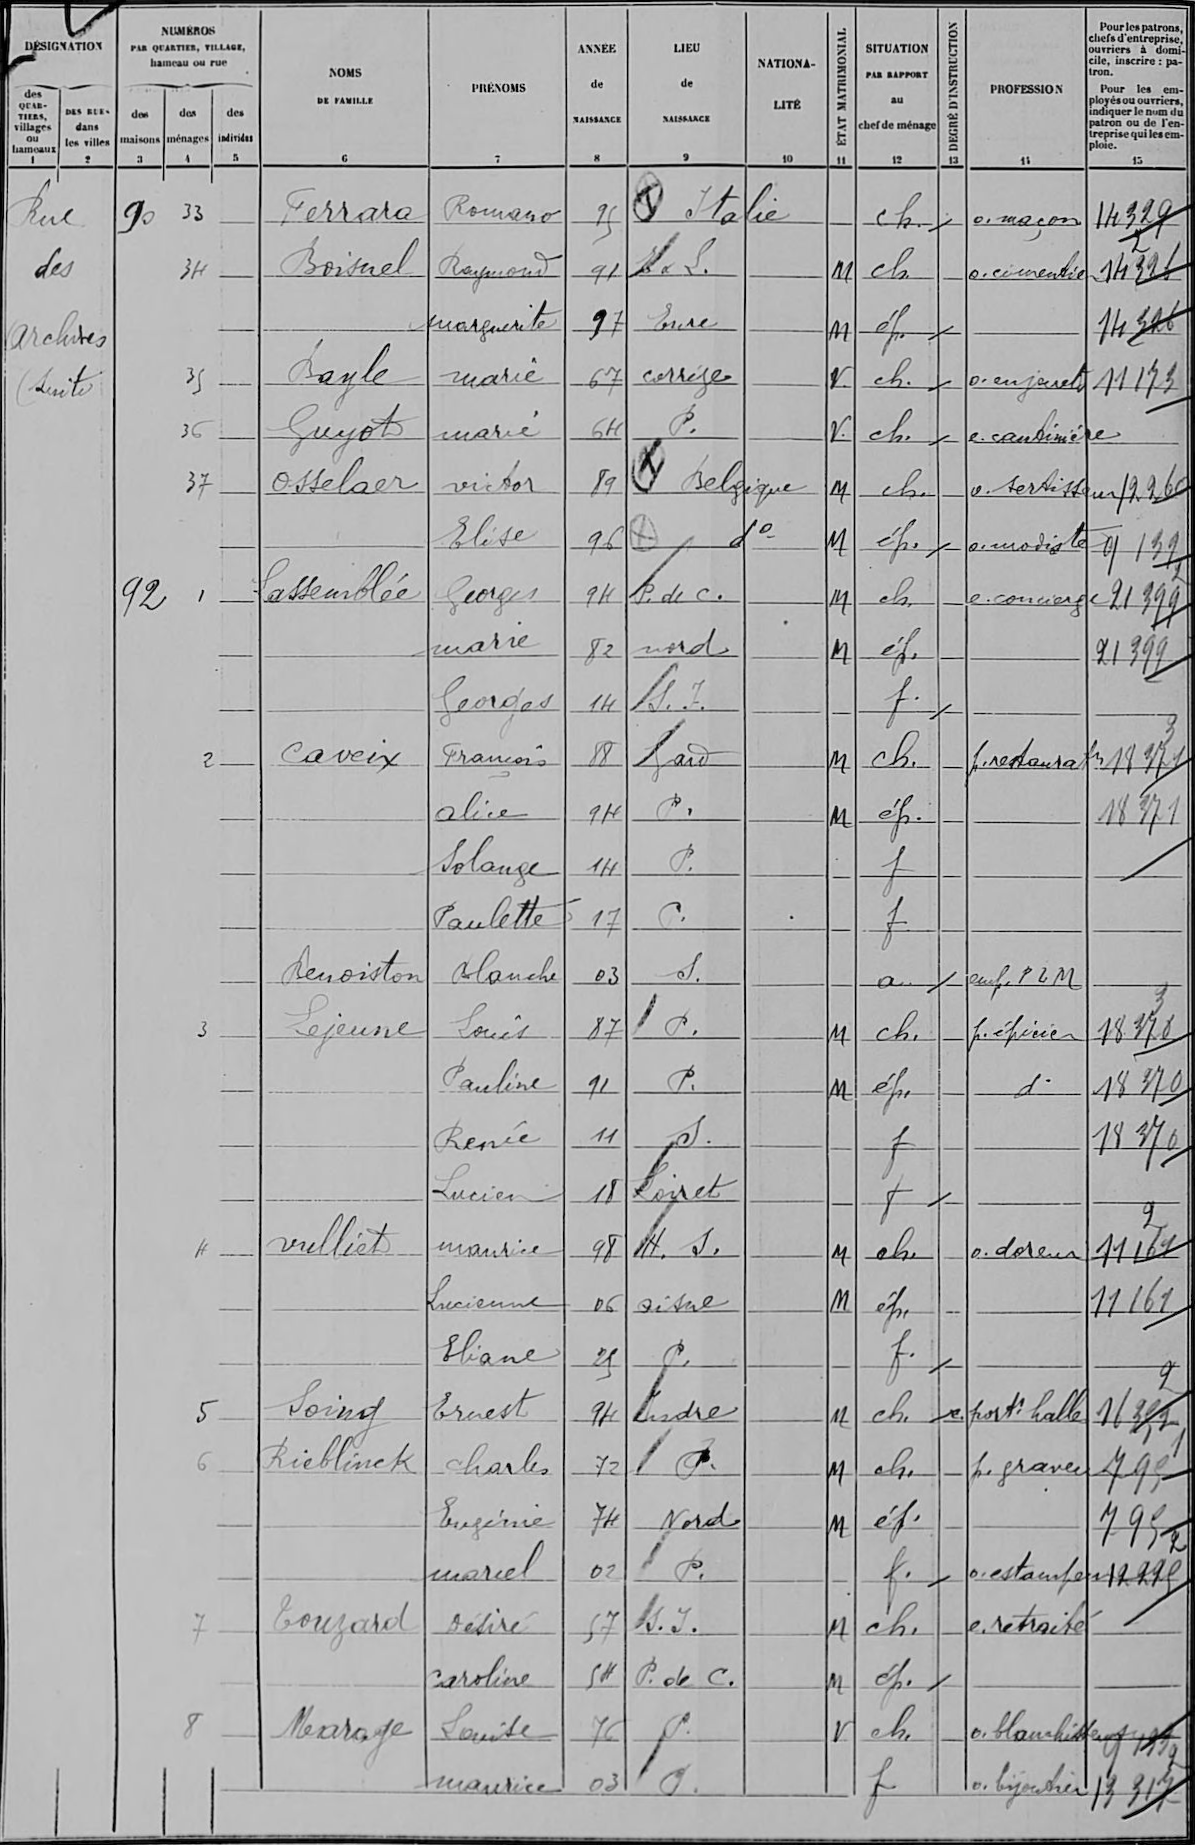Ferrara/Romano/95/Italie/¤/¤/ch /¤/o maçon/14329
Boisnel/Raymond/91/E & L /¤/M/ch /¤/o cimentier/14326
¤/Marguerite/97/eure/¤/M/ép /¤/¤/14326
Bayle/marie/67/corrèze/¤/V /ch /¤/o en jouets/11173
Guyot/Marie/64/P /¤/V /ch /¤/e cantinière/¤
Osselaer/victor/89/Belgique/¤/M/ch /¤/o Sertisseur/12260
¤/Elise/96/d°/¤/M/ép /¤/o modiste/9139
Lassemblée/Georges/94/P de C /¤/M/ch /¤/e concierge/21399
¤/marie/82/nord/¤/M/ép /¤/¤/21399
¤/Georges/14/S I /¤/¤/f /¤/¤/¤
Caveix/François/88/Gard/¤/M/ch /¤/p restaurant/18371
¤/Alice/94/P /¤/M/ép /¤/¤/18371
¤/Solange/14/P /¤/¤/f/¤/¤/¤
¤/Paulette/17/P /¤/¤/f/¤/¤/¤
Benoiston/Blanche/03/S /¤/¤/a /¤/emp PLM/¤
Lejeune/Louis/87/P /¤/M/ch /¤/p épicier/18370
¤/Pauline/91/P /¤/M/ép /¤/d°/18370
¤/Renée/11/S /¤/¤/f/¤/¤/18370
¤/Lucien/18/loiret/¤/¤/f/¤/¤/¤
vulliet/maurice/98/H S /¤/M/ch /¤/o doreur/11161
¤/Lucienne/06/Aisne/¤/M/ép /¤/¤/11161
¤/Eliane/25/P /¤/¤/f /¤/¤/¤
Soing/Ernest/94/Indre/¤/M/ch /¤/e portr halle/16352
Rieblinek/Charles/72/P /¤/M/ch /¤/p graveur/795
¤/Eugénie/74/Nord/¤/M/ép /¤/¤/795
¤/marcel/02/P /¤/¤/f /¤/o estampes/12225
Touzard/désiré/57/S I /¤/M/ch /¤/e retraité /¤
¤/caroline/54/P de C /¤/M/ép /¤/¤/¤
Marage/Louise/76/P /¤/V/ch /¤/o blanchisseuse/9133
¤/maurice/03/P /¤/¤/f/¤/o bijoutier/13317
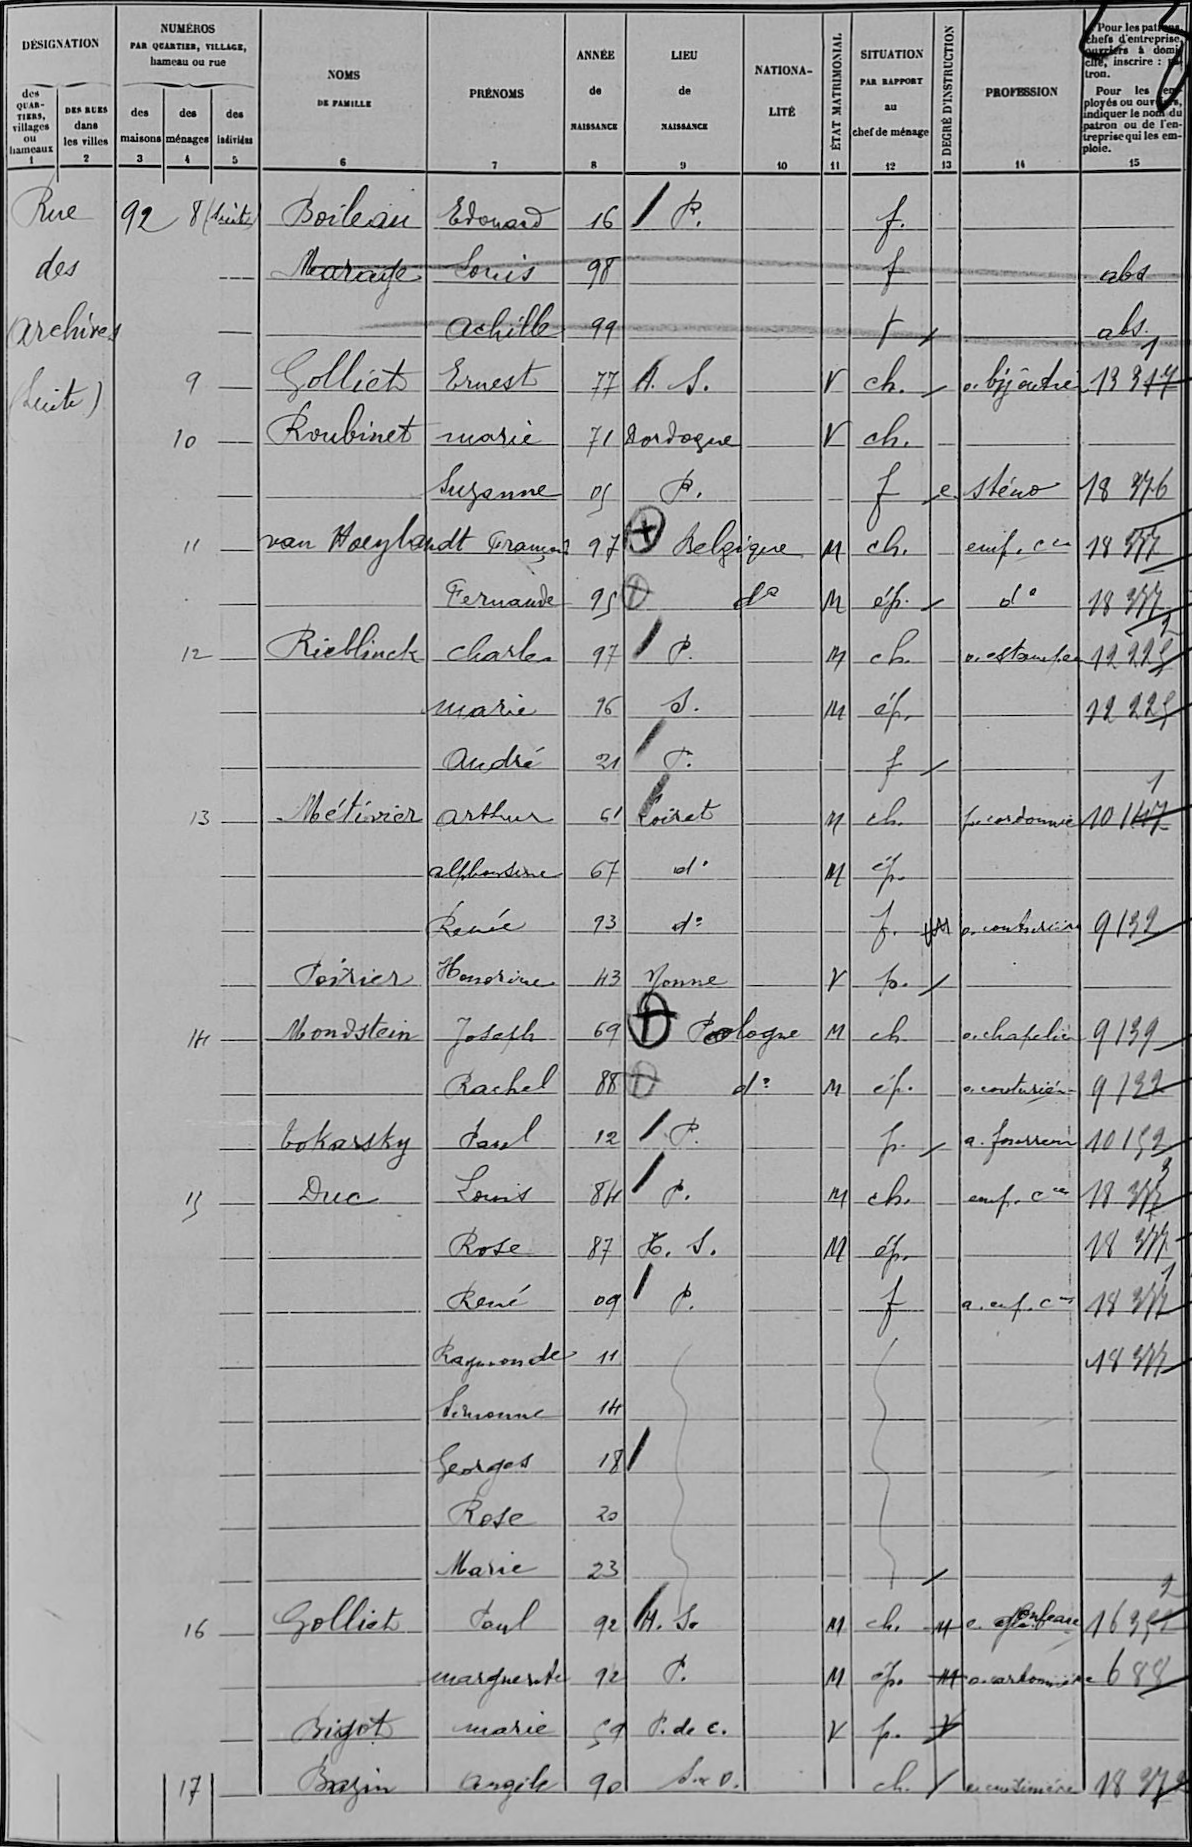Boileau/Edouard/16/P /¤/¤/f /¤/¤/¤
Marage/Louis/98/¤/¤/¤/f/¤/¤/abs
¤/Achille/99/¤/¤/¤/f/¤/¤/abs
Golliet/Ernest/77/H S /¤/V/ch /¤/o bijoutier/13317
Roubinet/marie/71/Dordogne/¤/V/ch /¤/¤/¤
¤/Suzanne/05/P/¤/¤/f/¤/e sténo/18376
van Hoeybandt/François/97/Belgique/¤/M/ch /¤/emp Cce/18377
¤/Fernande/95/¤/d°/M/ép /¤/d°/18377
Rieblinck/Charles/97/P /¤/M/ch /¤/o estampes/12225
¤/Marie/96/S /¤/M/ép /¤/¤/12225
¤/André/21/P /¤/¤/f/¤/¤/¤
Métivier/Arthur/61/Loiret/¤/M/ch /¤/p cordonnier/10147
¤/Alphonsine/67/d°/¤/M/ép /¤/¤/¤
¤/Renée/93/D°/¤/¤/f/¤/o couturière/9132
Poirier/Honorine/43/Yonne/¤/V/p /¤/¤/¤
Mondstein/Joseph/69/Pologne/¤/M/ch /¤/o chapelier/9139
¤/Rachel/88/d°/¤/M/ép /¤/o couturière/9132
Tokarsky/Paul/12/P /¤/¤/f /¤/a fourreur/10159
Duc/Louis/84/P /¤/M/ch /¤/emp Cce/18377
¤/Rose/87/H S /¤/M/ép /¤/¤/18377
¤/René/09/P /¤/¤/f/¤/a empl Cce/18377
¤/Raymonde/11/¤/¤/¤/¤/¤/¤/18377
¤/Simonne/14/¤/¤/¤/¤/¤/¤/¤
¤/Georges/19/¤/¤/¤/¤/¤/¤/¤
¤/Rose/20/¤/¤/¤/¤/¤/¤/¤
¤/Marie/23/¤/¤/¤/¤/¤/¤/¤
Golliet/Paul/92/H S /¤/M/ch /M/e Gonfaie/16352
¤/Marguerite/92/P /¤/M/ép /M/o cartonière/688
Bigot/Marie/59/P de C /¤/V/p /V/¤/¤
Bazin/Angèle/90/S & O /¤/¤/ch /¤/e cuisinière/18372